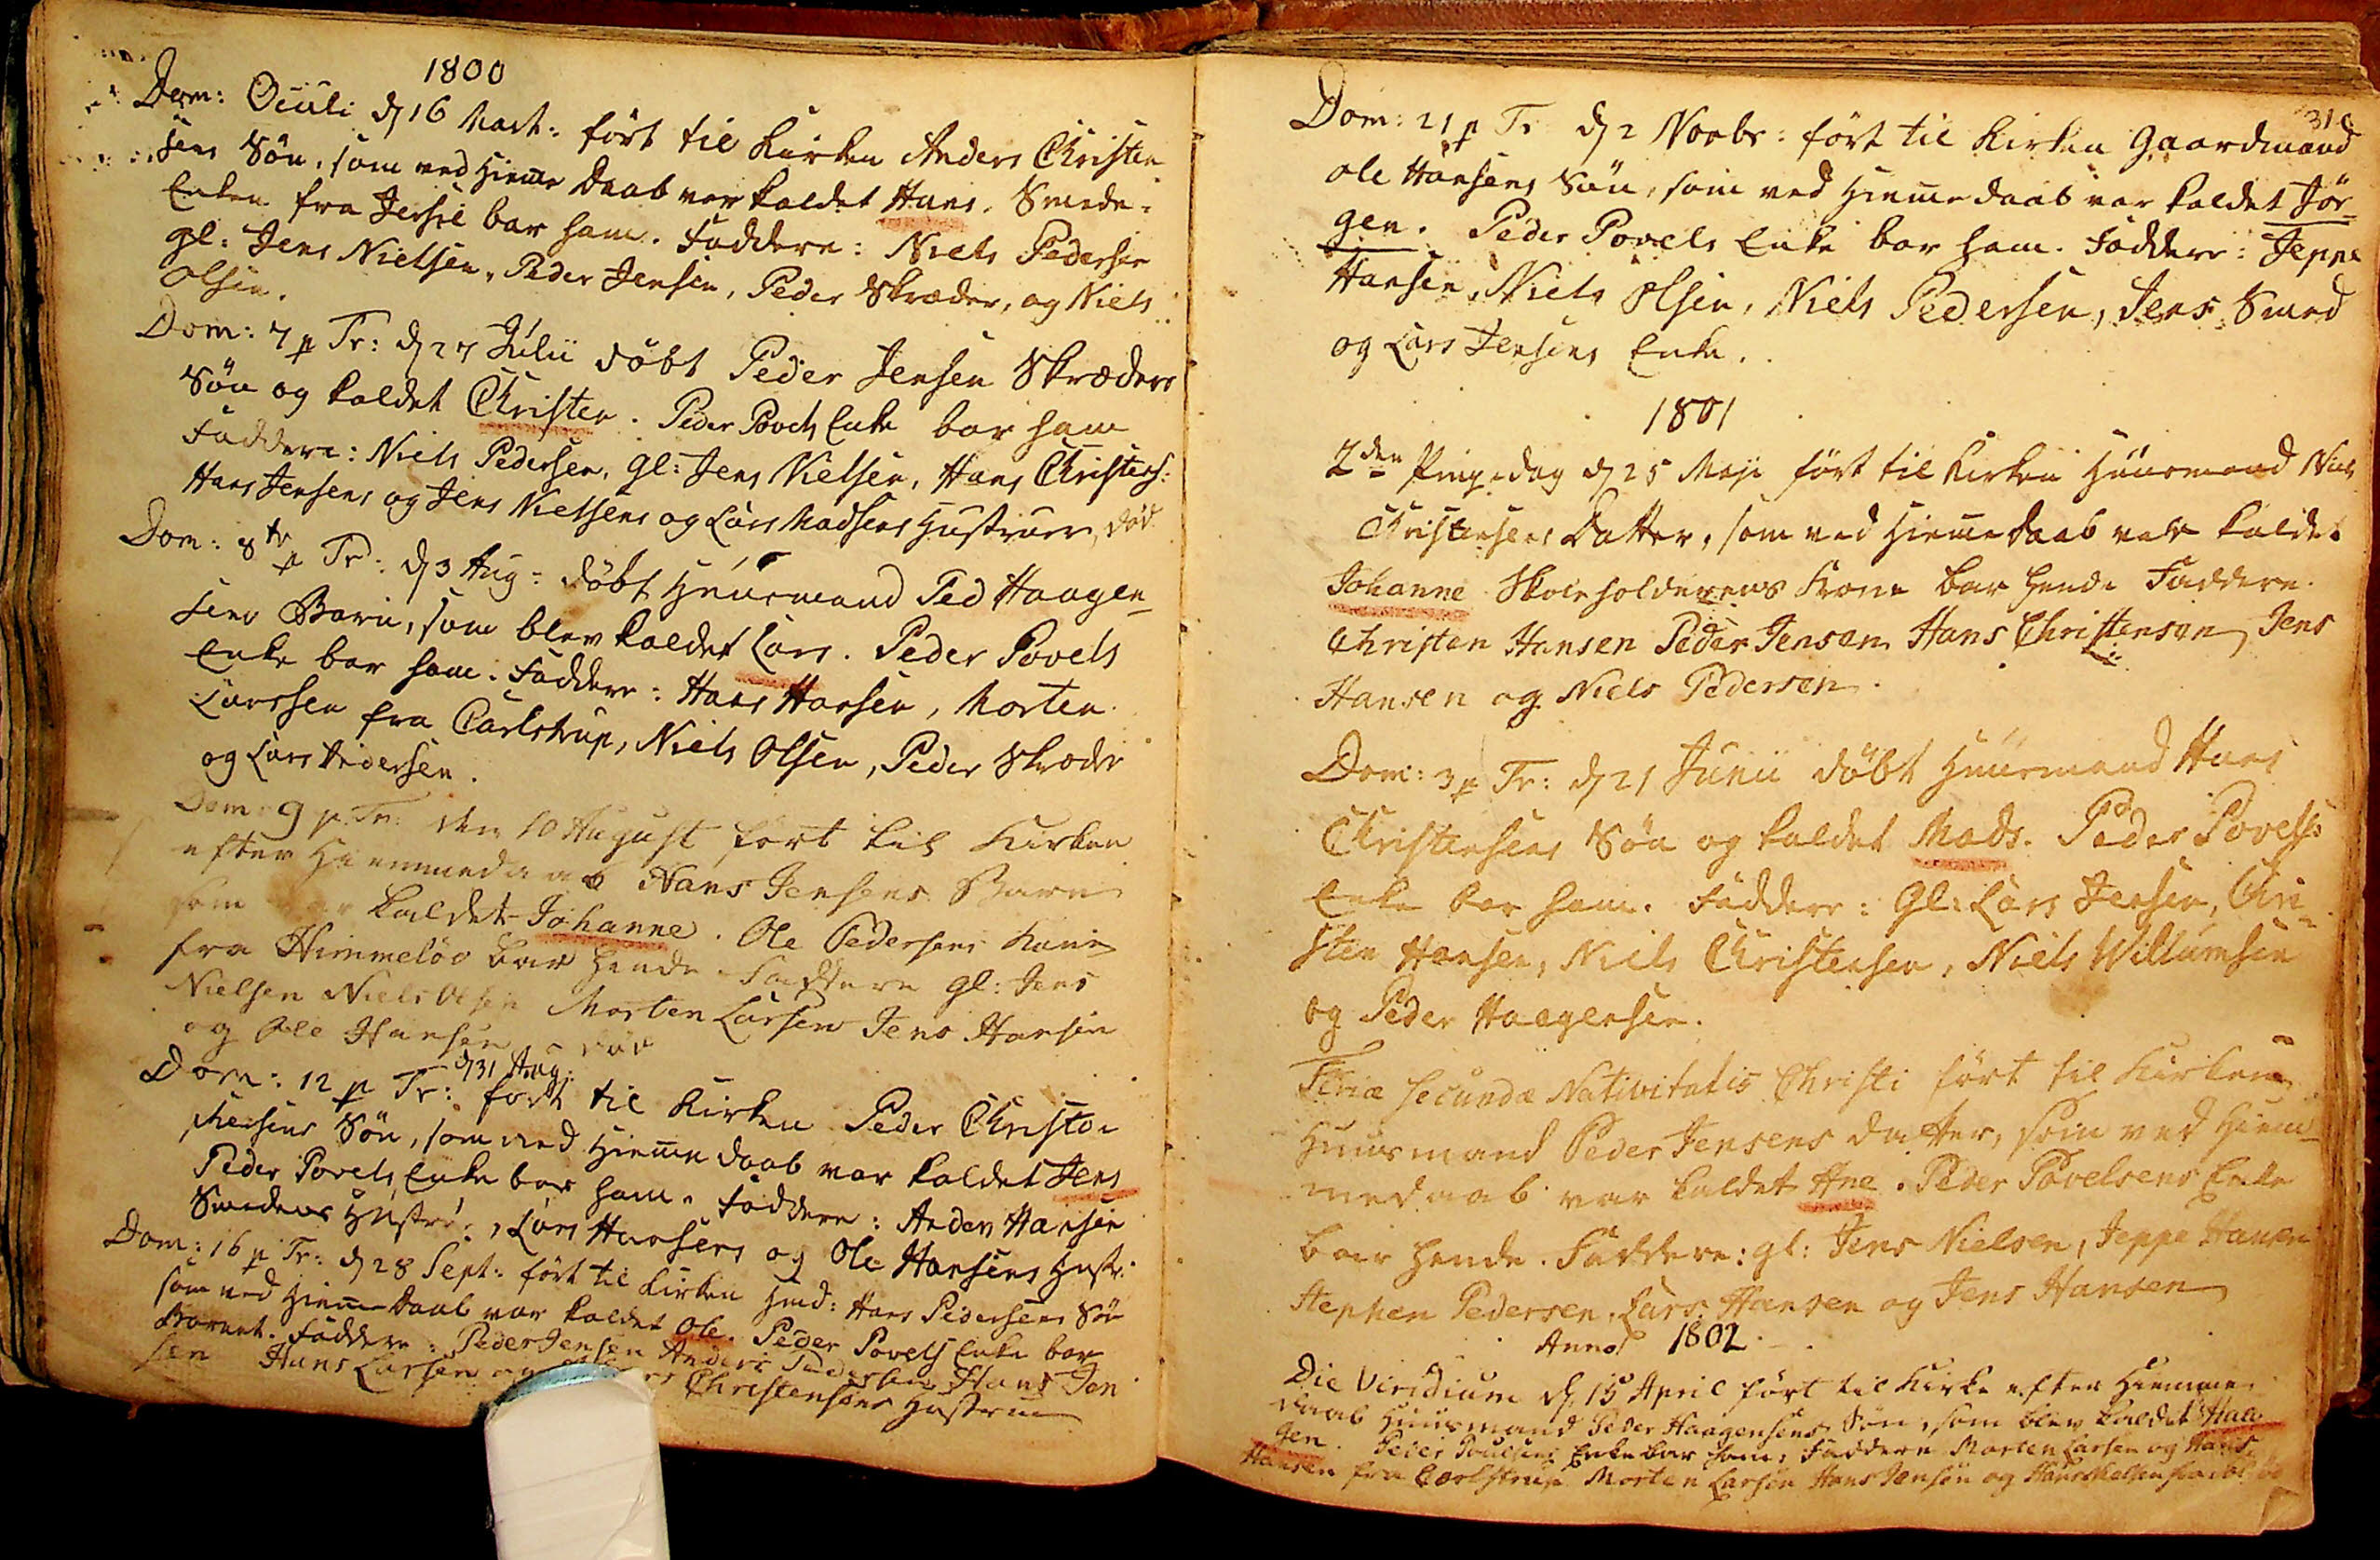1800
Dom: Oculi d 16 Mart: ført til Kirken Anders Christen
sens Søn, som ved Hieme Daab var kaldet Hans. Smede¬
Enken fra Jersie bar ham. Faddere: Niels Pedersen
gl: Jens Nielsen, Peder Jensen, Peder Skræder, og Niels
Olsen.
Dom: 7 p Tr: d 27 Julii døbt Peder Jensen Skræders
Søn og kaldet Christen. Peder Povels Enke bar ham
Faddere: Niels Pedersen, gl: Jens Nielsen, Hans Christens:
Hans Jensens og Jens Nielsens og Lars Madsens Hustruer, død
Dom: 8te p Tr: d 3 Aug: døbt Huusmand Ped Haagen
sens Barn, som blev kaldet Lars. Peder Povels
Enke bar ham. Faddere: Hans Hansen, Morten
Larssen fra Carlstrup, Niels Olsen, Peder Skræder
og Lars Andersen.
Dom: 9 p Tr: den 10 August ført til Kirken
efter Hiemmedaab Hans Jensens Barn
som var kaldet Johanne. Ole Pedersens Kone
fra Himmeløv bar hende Faddere gl: Jens
Nielsen Niels Olsen Morten Larsen Jens Hansen
og Ole Hansen død
d 31 Aug:
Dom: 12 p Tr: ført til Kirken Peder Christo¬
phersens Søn, som ved Hiemedaab var kaldet Jens
Peder Povels Enke bar ham. Faddere: Anders Hansen
Smedens Hustrue, Lars Hansens og Ole Hansens Hustr:
Dom: 16 p Tr: d 28 Sept: ført til Kirken Hmd: Hans Pedersens Søn
som ved hjemeDaab var kaldet Ole. Peder Povels Enke bar
Barnet. Faddere: Peder Jensen Anders Pedersen Hans Jen
sen Hans Larsen og Anders Christensens Hustrue
31.
Dom: 21 p Tr: d 2 Novbr: ført til Kirken Gaardmand
Ole Hansens Søn, som ved Hiemedaab var kaldet Jør¬
gen. Peder Povels Enke bar ham. Faddere: Jeppe
Hansen, Niels Olsen, Niels Pedersen, Jens Smed
og Lars Jensens Enke.
1801
2den Pinzedag d 25 Maji ført til Kirken Huusmand Niels
Christensens Datter, som ved HiemeDaab var kaldet
Johanne. Skoleholderens Kone bar hende Faddere:
Christen Hansen Peder Jensen Hans Christensen Jens
Hansen og Niels Pedersen.
Dom: 3 p Tr: d 21 Junii døbt Huusmand Hans
Christensens Søn og kaldet Mads. Peder Povels
Enke bar ham. Faddere: Gl: Lars Jensen, Chri¬
sten Hansen, Niels Christensen, Niels Willumsen
og Peder Haagensen.
Feria secundæ Nativitatis Christi ført til Kirken
Huusmand Peder Jensens Datter, som ved Hiem¬
medaab var kaldet Ane. Peder Povels Enke
bar hende. Faddere: gl: Jens Nielsen, Jeppe Hansen
Stephen Pedersen, Lars Hansen og Jens Hansen.
Anno 1802.
Die Viridium d: 15 April ført til Kirke efter Hiemme
daab Huusmand Peder Haagensens Søn, som blev kaldet Haa
gen. Peder Pouelsens Enke bar ham: Faddere: Morten Larsen og Hans
Hansen fra Carlstrup Morten Larsen Hans Jensen og Hans Nielsen fra Solrød
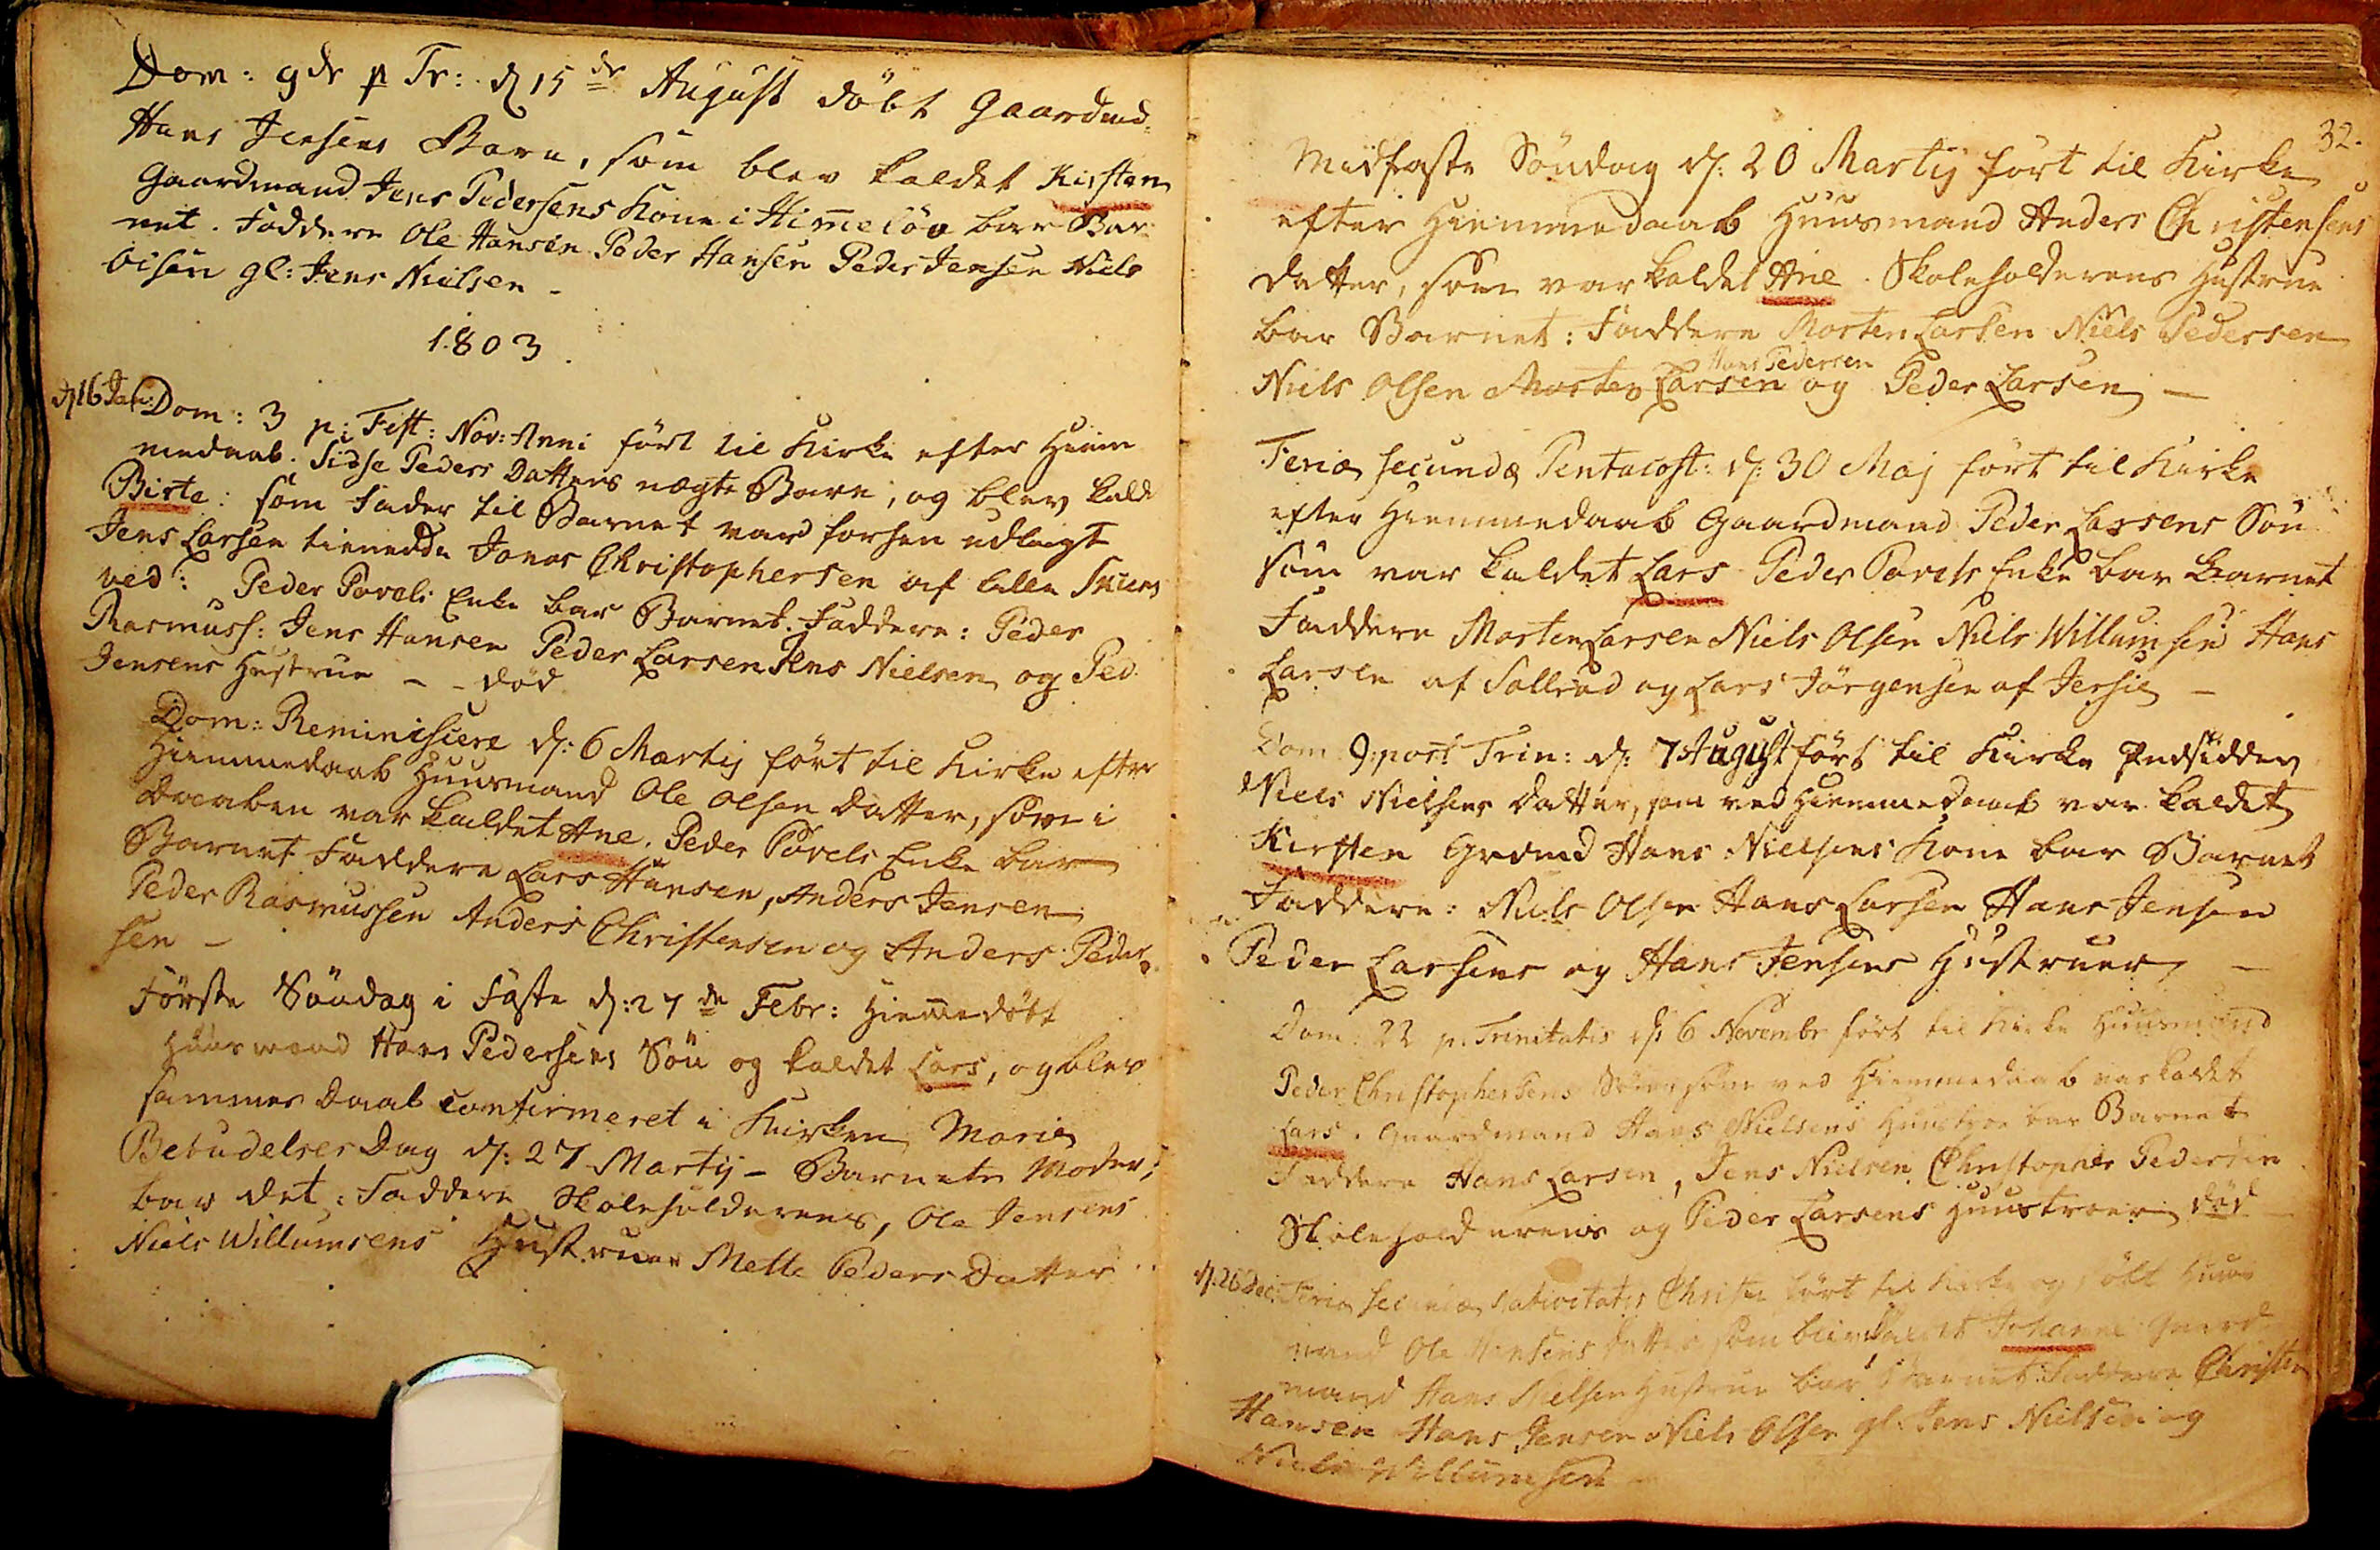Dom: 9de p Tr: d 15de August døbt Gaardmd
Hans Jensens Barn, som blev kaldet Kirsten.
Gaardmand Jens Pedersens Kone i Himeløv bar Bar¬
net. Faddere Ole Hansen Peder Hansen Peder Jensen Niels
Olsen gl: Jens Nielsen.
1803.
d 16 Jan: Dom: 3 p: Fest: Nov: Anni ført til Kirke efter Hiem
medaab Sidse Peders Datters uægte Barn, og blev kaldt
Birte: som Fader til Barnet var forhen udlagt
Jens Larsen tienende Jonas Christophersen af lille Skiens
ved: Peder Povels Enke bar Barnet. Faddere: Peder
Rasmuss: Jens Hansen Peder Larsen Jens Nielsen og Ped.
Jensens Hustrue - - død.
Dom: Reminiscere d: 6 Martij ført til kirke efter
Hiemmedaab Huusmand Ole Olsen Datter, som i
Daaben var kaldet Ane. Peder Povels Enke bar
Barnet Faddere Lars Hansen, Anders Jensen
Peder Rasmussen Anders Christensen og Anders Peder¬
sen
Første Søndag i Faste d: 27de Febr: Hiemmedøbt
Huusmand Hans Pedersens Søn og kaldet Lars, og blev
sammes Daab confirmeret i Kirken Marie
Bebudelses Dag d: 27 Martij -- Barnets Moder
bar det: Faddere Skoleholderens, Ole Jensens
Niels Willumsens Hustruer Mette Peders Datter.
32.
Midfaste Søndag d: 20 Martij ført til Kirke
efter Hiemmedaab Huusmand Anders Christensens
datter, som var kaldet Ane. Skoleholderens Hustrue
bar Barnet: Faddere Morten Larsen Niels Pedersen
Hans Pedersen
Niels Olsen Morten Larsen og Peder Larsen
Feria secundæ Pentacost: d: 30 Maj ført til Kirke
efter Hiemmedaab Gaardmand Peder Larsens Søn
som var kaldet Lars. Peder Povels Enke bar Barnet
Faddere Morten Larsen Niels Olsen Niels Willumsen Hans
Larsen af Sollrød og Lars Jørgensen af Jersie
Dom: 9: post Trin: d: 7 August ført til Kirke Indsidder
Niels Nielsens Datter, som ved Hiemmedaab var kaldet
Kirsten Grdmd Hans Nielsens Kone bar Barnet
Faddere: Niels Olsen Hans Larsen Hans Jensen
Peder Larsens og Hans Jensens Hustruer
Dom: 22 p: Trinitatis d: 6 Novembr ført til Kirke Huusmand
Peder Christophersens Søn, som ved Hiemmedaab var kaldet
Lars. Gaardmand Hans Nielsens Huustroe bar Barnet
Faddere Hans Larsen, Jens Nielsen Christopher Pedersen
Skoleholderens og Peder Larsens Huustroer død
d: 26 Dec: Feria secundæ Nativitatis Christi ført til Kirke og døbt Huus
mand Ole Hansens Datter, som blev kaldet Johanne. Gaard
mand Hans Nielsen Hustrue bar Barnet: Faddere Christen
Hansen Hans Jensen Niels Olsen gl: Jens Nielsen og
Niels Willumsen